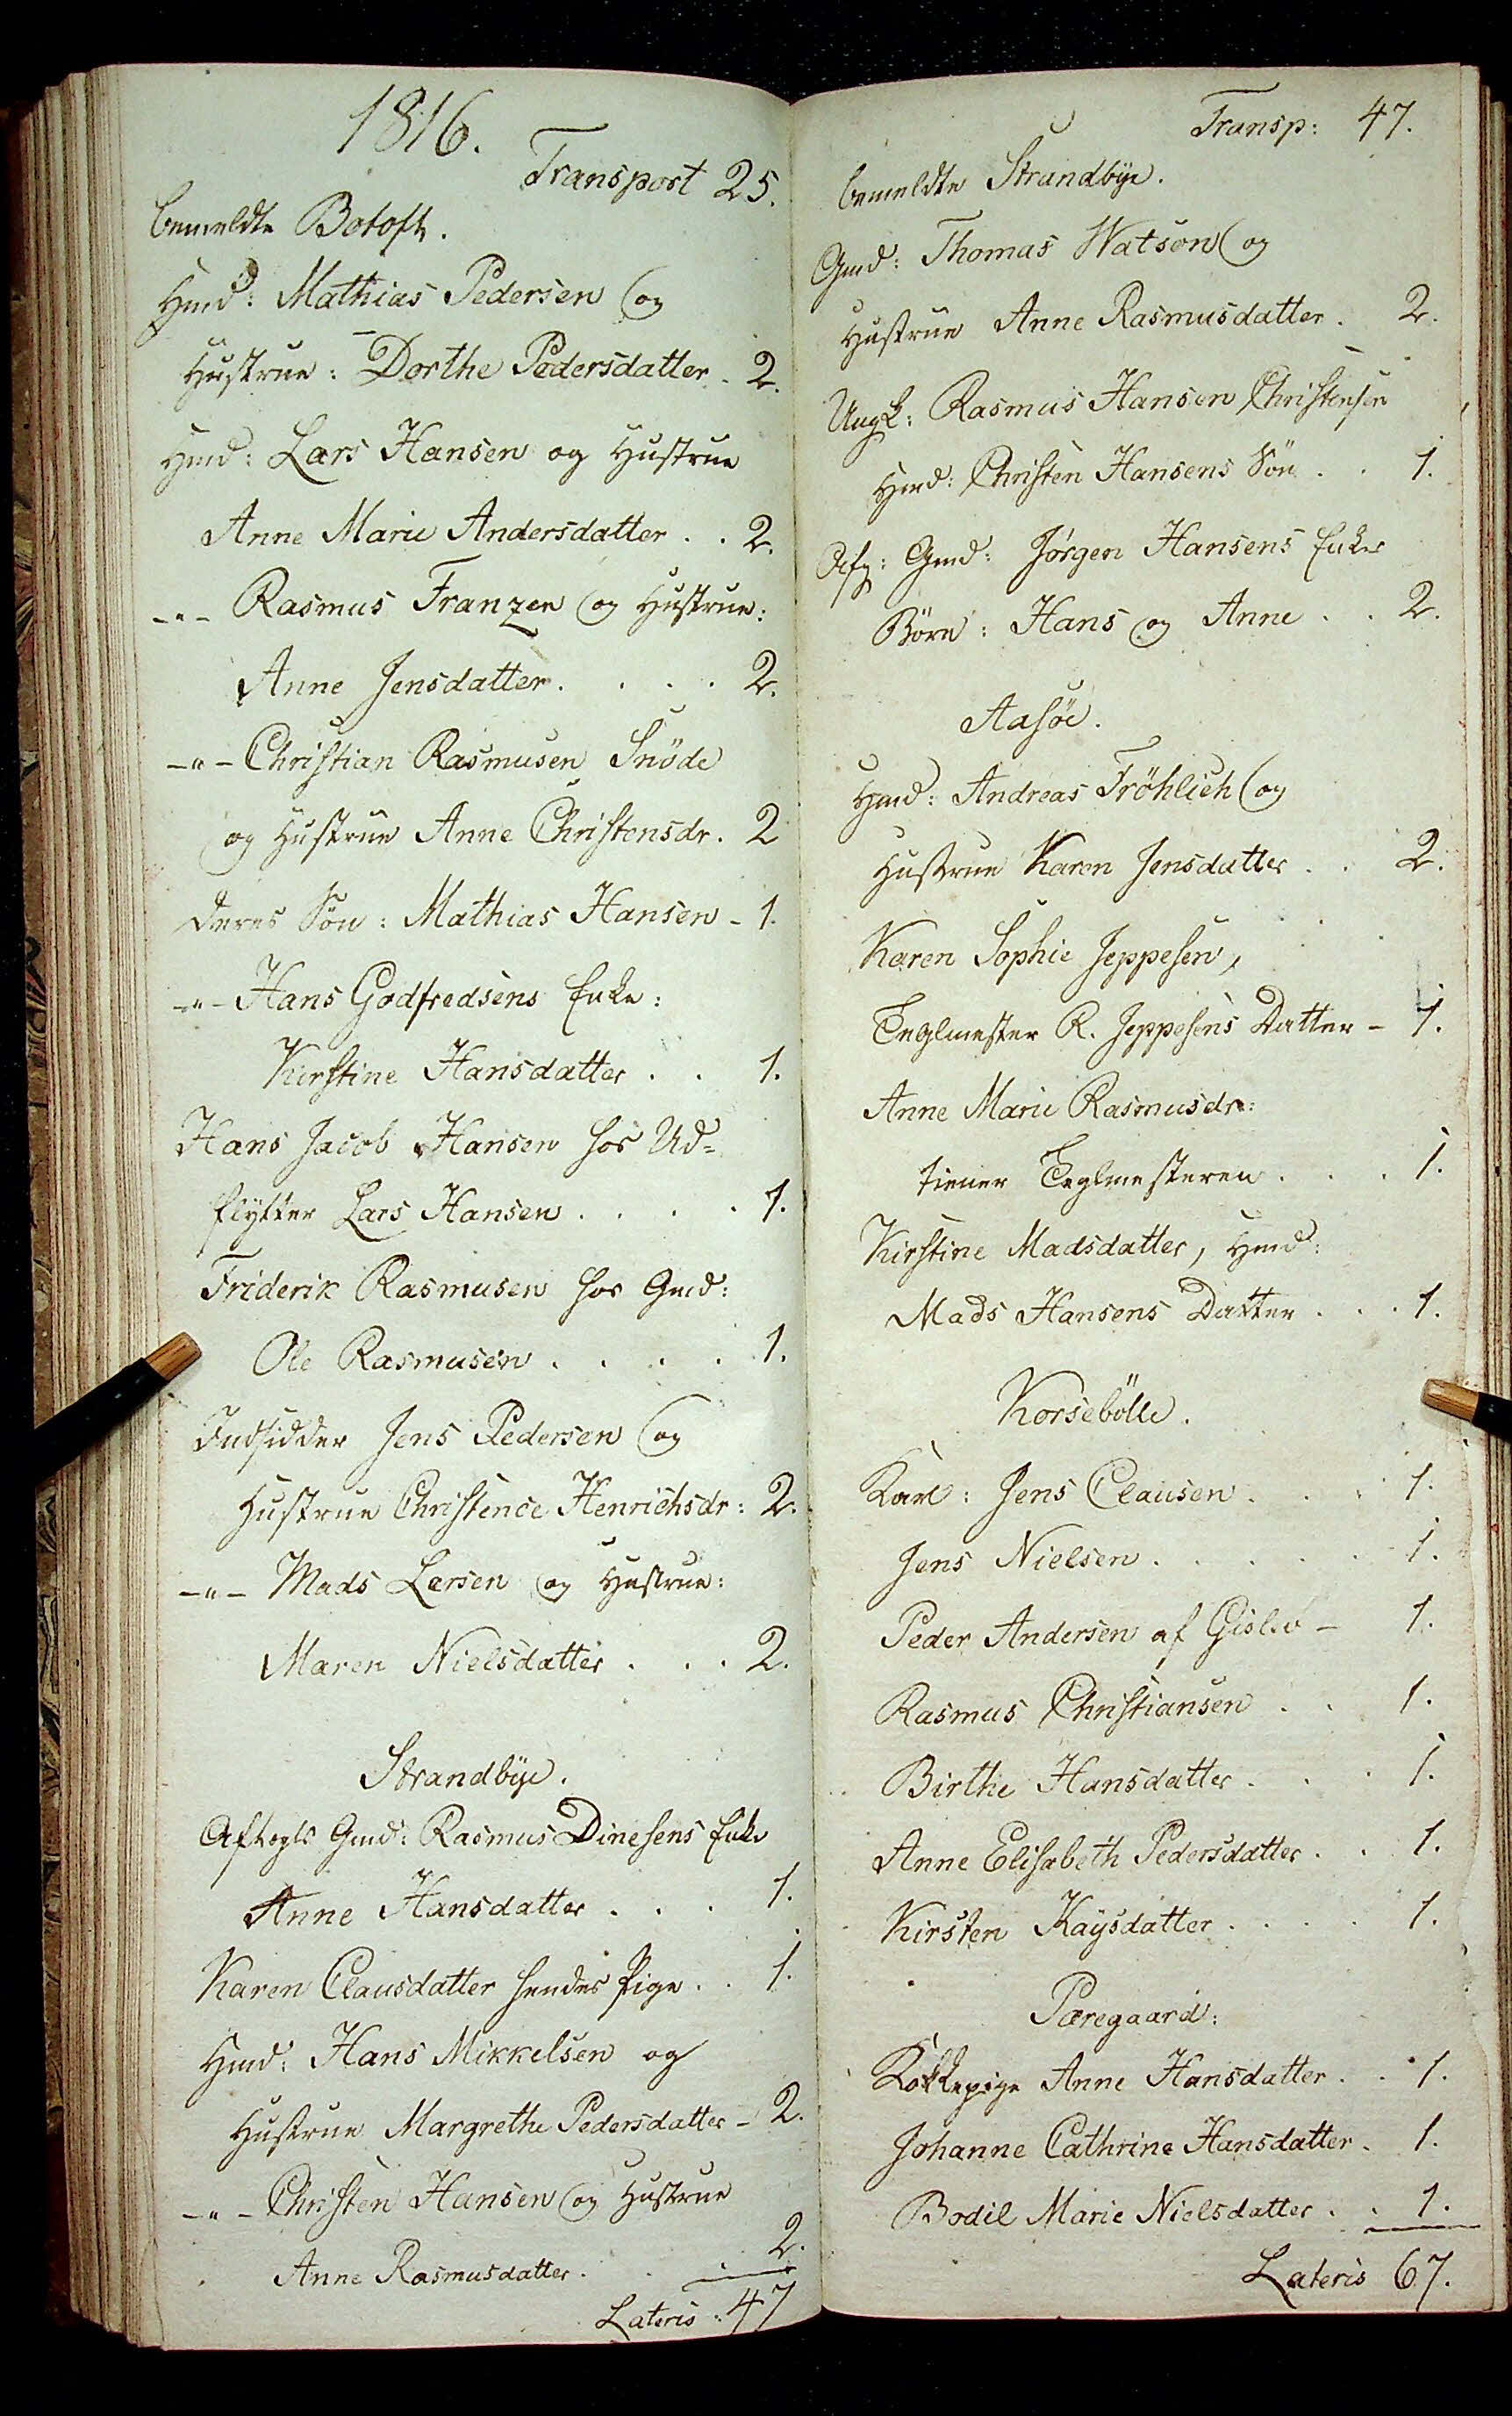1816.
Transport 25.
bemeldte Botoft.
Hmd: Mathias Pedersen og
Hustrue: Dorthe Peitersdatter . 2.
Hmd: Lars Hansen og Hustrue
Anne Marie Andersdatter . . 2.
-"- Rasmus Franzen og Hustrue:
Anne Jensdatter . . . . 2.
-"- Christian Rasmusen Snøde
og Hustrue Anne Christensdr . 2
deres Søn: Mathias Hansen - 1.
-"- Hans Godfredsens Enke:
Kirstine Hansdatter . . 1.
Hans Jacob Hansen hos Ud¬
flytter Lars Hansen . . . . 1.
Friderik Rasmusen hos Gmd:
Ole Rasmusen . . . . 1.
Indsidder Jens Pedersen og
Hustrue Christence Henrichsdr: 2.
-"- Mads Larsen og Hustrue:
Maren Nielsdatter . . . 2.
Strandbye.
Aftægts Gmd: Rasmus Dinesens Enke
Anne Hansdatter . . . 1.
Karen Clausdatter hendes Pige . . 1.
Hmd: Hans Mikkelsen og
Hustrue Margrethe Pedersdatter - 2.
-"- Christen Hansen og Hustrue
Anne Rasmusdatter . . . 2.
Lateris: 47
Transp: 47.
bemeldte Stranbye.
Gmd: Thomas Watson og
Hustrue Anne Rasmusdatter . 2.
Ungk: Rasmus Hansen Christensen
Hmd: Christen Hansens Søn . . 1.
Oefg## Gmd: Jørgen Hansens Enkes
Børn: Hans og Anne . . 2.
Aasøe.
Hmd: Andreas Frøhlich og
Hustrue Karen Jensdatter . . 2.
Karen Sophie Jeppesen,
Teglmester R. Jeppesens Datter - 1.
Anne Marie Rasmusdr:
tiener Teglmesteren . . . 1.
Kirstine Madsdatter, Hmd:
Mads Hansens Datter . . . 1.
Korsebølle.
Karl: Jens Clausen . . . 1.
Jens Nielsen . . . . . 1.
Peder Andersen af Gislev - 1.
Rasmus Christiansen . . 1.
Birthe Hansdatter . . . 1.
Anne Elisabeth Pedersdatter . . 1.
Kirsten Madsdatter . . . . 1.
Pæregaard:
Kokkepige Anne Hansdatter . . 1.
Johanne Cathrine Hansdatter . 1.
Bodil Marie Nielsdatter . . 1.
Lateris 67.
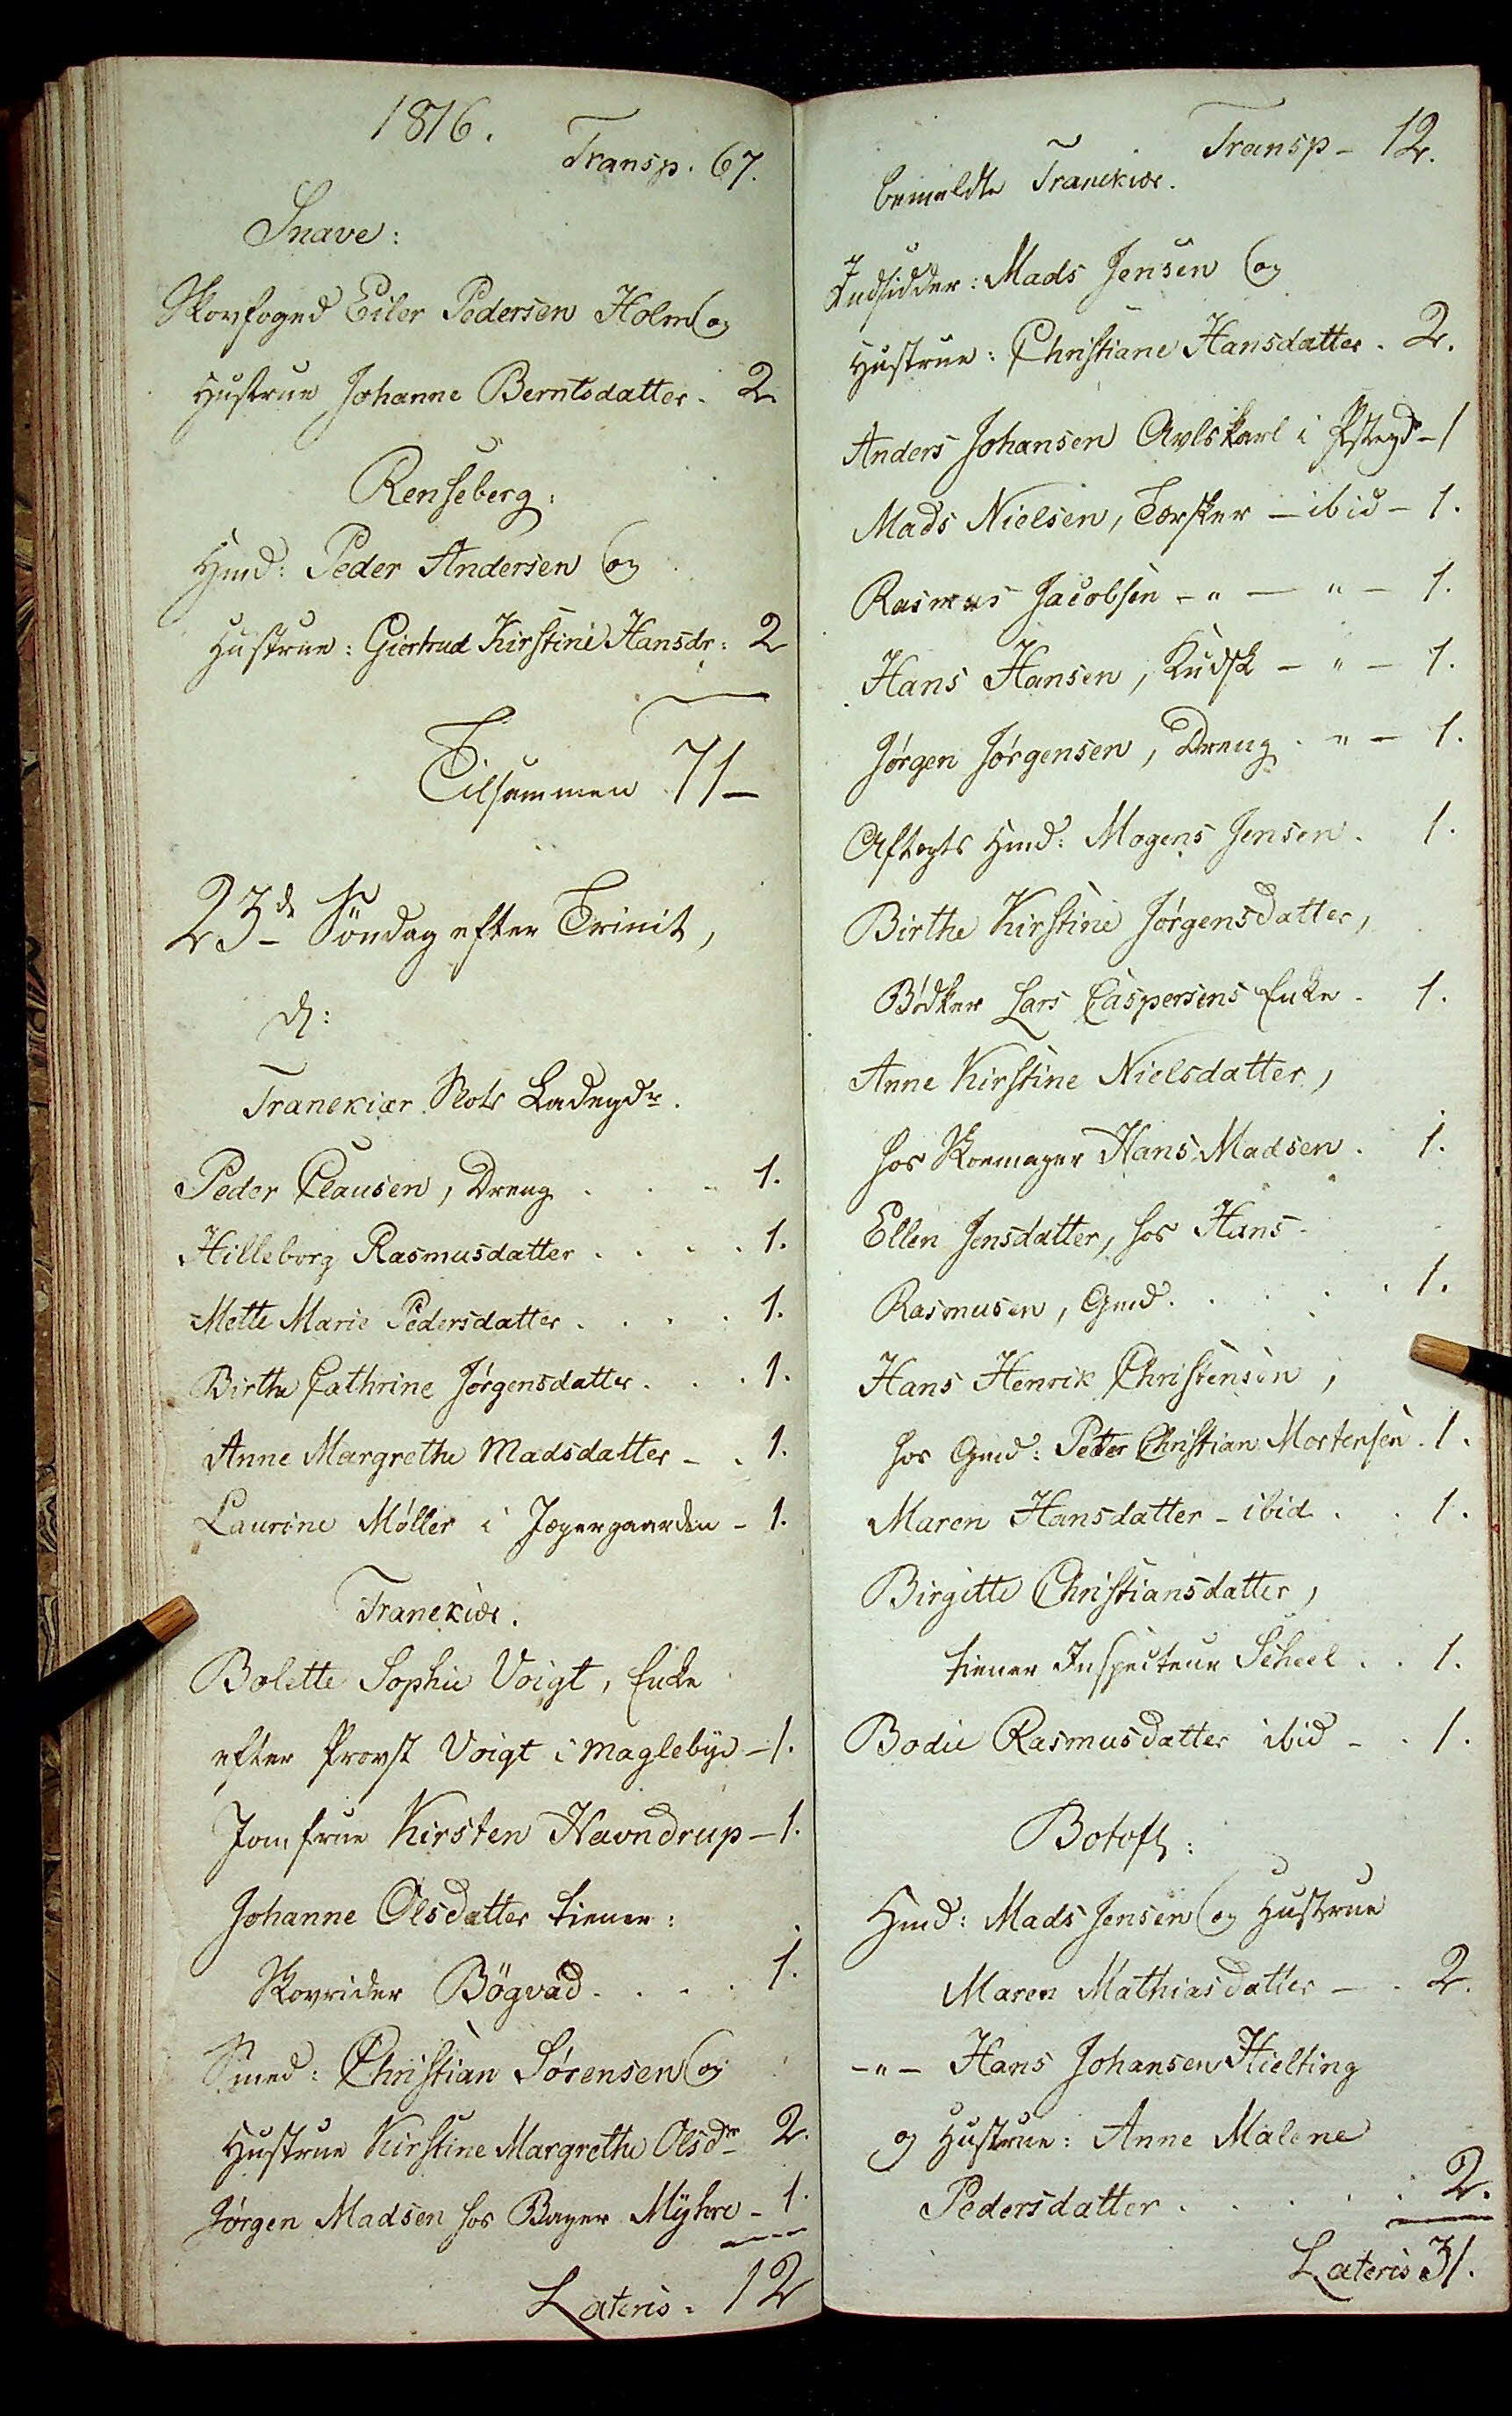1816.
Transp. 67.
Sna ve:
Skoefoged Eiler Pedersen Holm og
Hustrue Johanne Berntsdatter . 2.
Renseberg:
Hmd: Peder Andersen og
Hustrue: Giertrud Kirstine Hansdr : 2
Tilsammen 71-
23de Søndag efter Trinit,
d:
Tranekiær Slots Ladegdr.
Peder Clausen, Dreng . . - 1.
Hilleborg Rasmusdatter . . . . 1.
Mette Marie Pedersdatter . . . . 1.
Birthe Cathrine Jørgensdatter . . . 1.
Anne Margrethe Madsdatter - . 1.
Laurine Møller i Jægergaarden - 1.
Tranekiær.
Bolette Sophie Voigt. Enke
efter Provst Voigt i Maglebye - 1.
Jomfrue Kirsten Havndrup - 1.
Johanne Olsdatter tiener:
Skovrider Bøgvad . . . . 1.
Smed: Christian Sørensen og
Hustrue Kirstine Margrethe Olsdr 2.
Jørgen Madsen hos Bager Muhre - 1.
Lateris = 12
Transp - 12.
bemeldte Tranekiær.
Indsidder Mads Jensen og
Hustrue: Christiane Hansdatter . 2.
Anders Johansen Avlskarl i Pstegdr - 1
Mads Nielsen, Tærsker - i-bid - 1.
Rasmus Jacobsen -"- -"- 1.
Hans Hansen, Kudsk -"- 1.
Jørgen Jørgensen, Dreng -"- 1.
Aftægts Hmd: Mogens Jensen . 1
Birthe Kirstine Jørgensdatter,
Bødker Lars Caspersens Enke . 1.
Anne Kirstine Nielsdatter,
hos Skoemager Hans Madsen . 1.
Ellen Jensdatter, hos Hans
Rasmusen, Gmd . . . . . 1.
Hans Henrik Christensen,
hos Gmd: Peter Christian Mortensen . 1.
Maren Hansdatter - ibid . . 1
Birgitte Christiansdatter,
tiener Inspecteur Scheel . . 1.
Bodil Rasmusdatter ibid - . 1.
Botoft
Hmd: Mads Jensen og Hustrue
Maren Mathiasdatter - . 2.
-"- Hans Johansen Hielting
og Hustrue: Anne Malene
Pedersdatter . . . . . 2.
Lateris 31